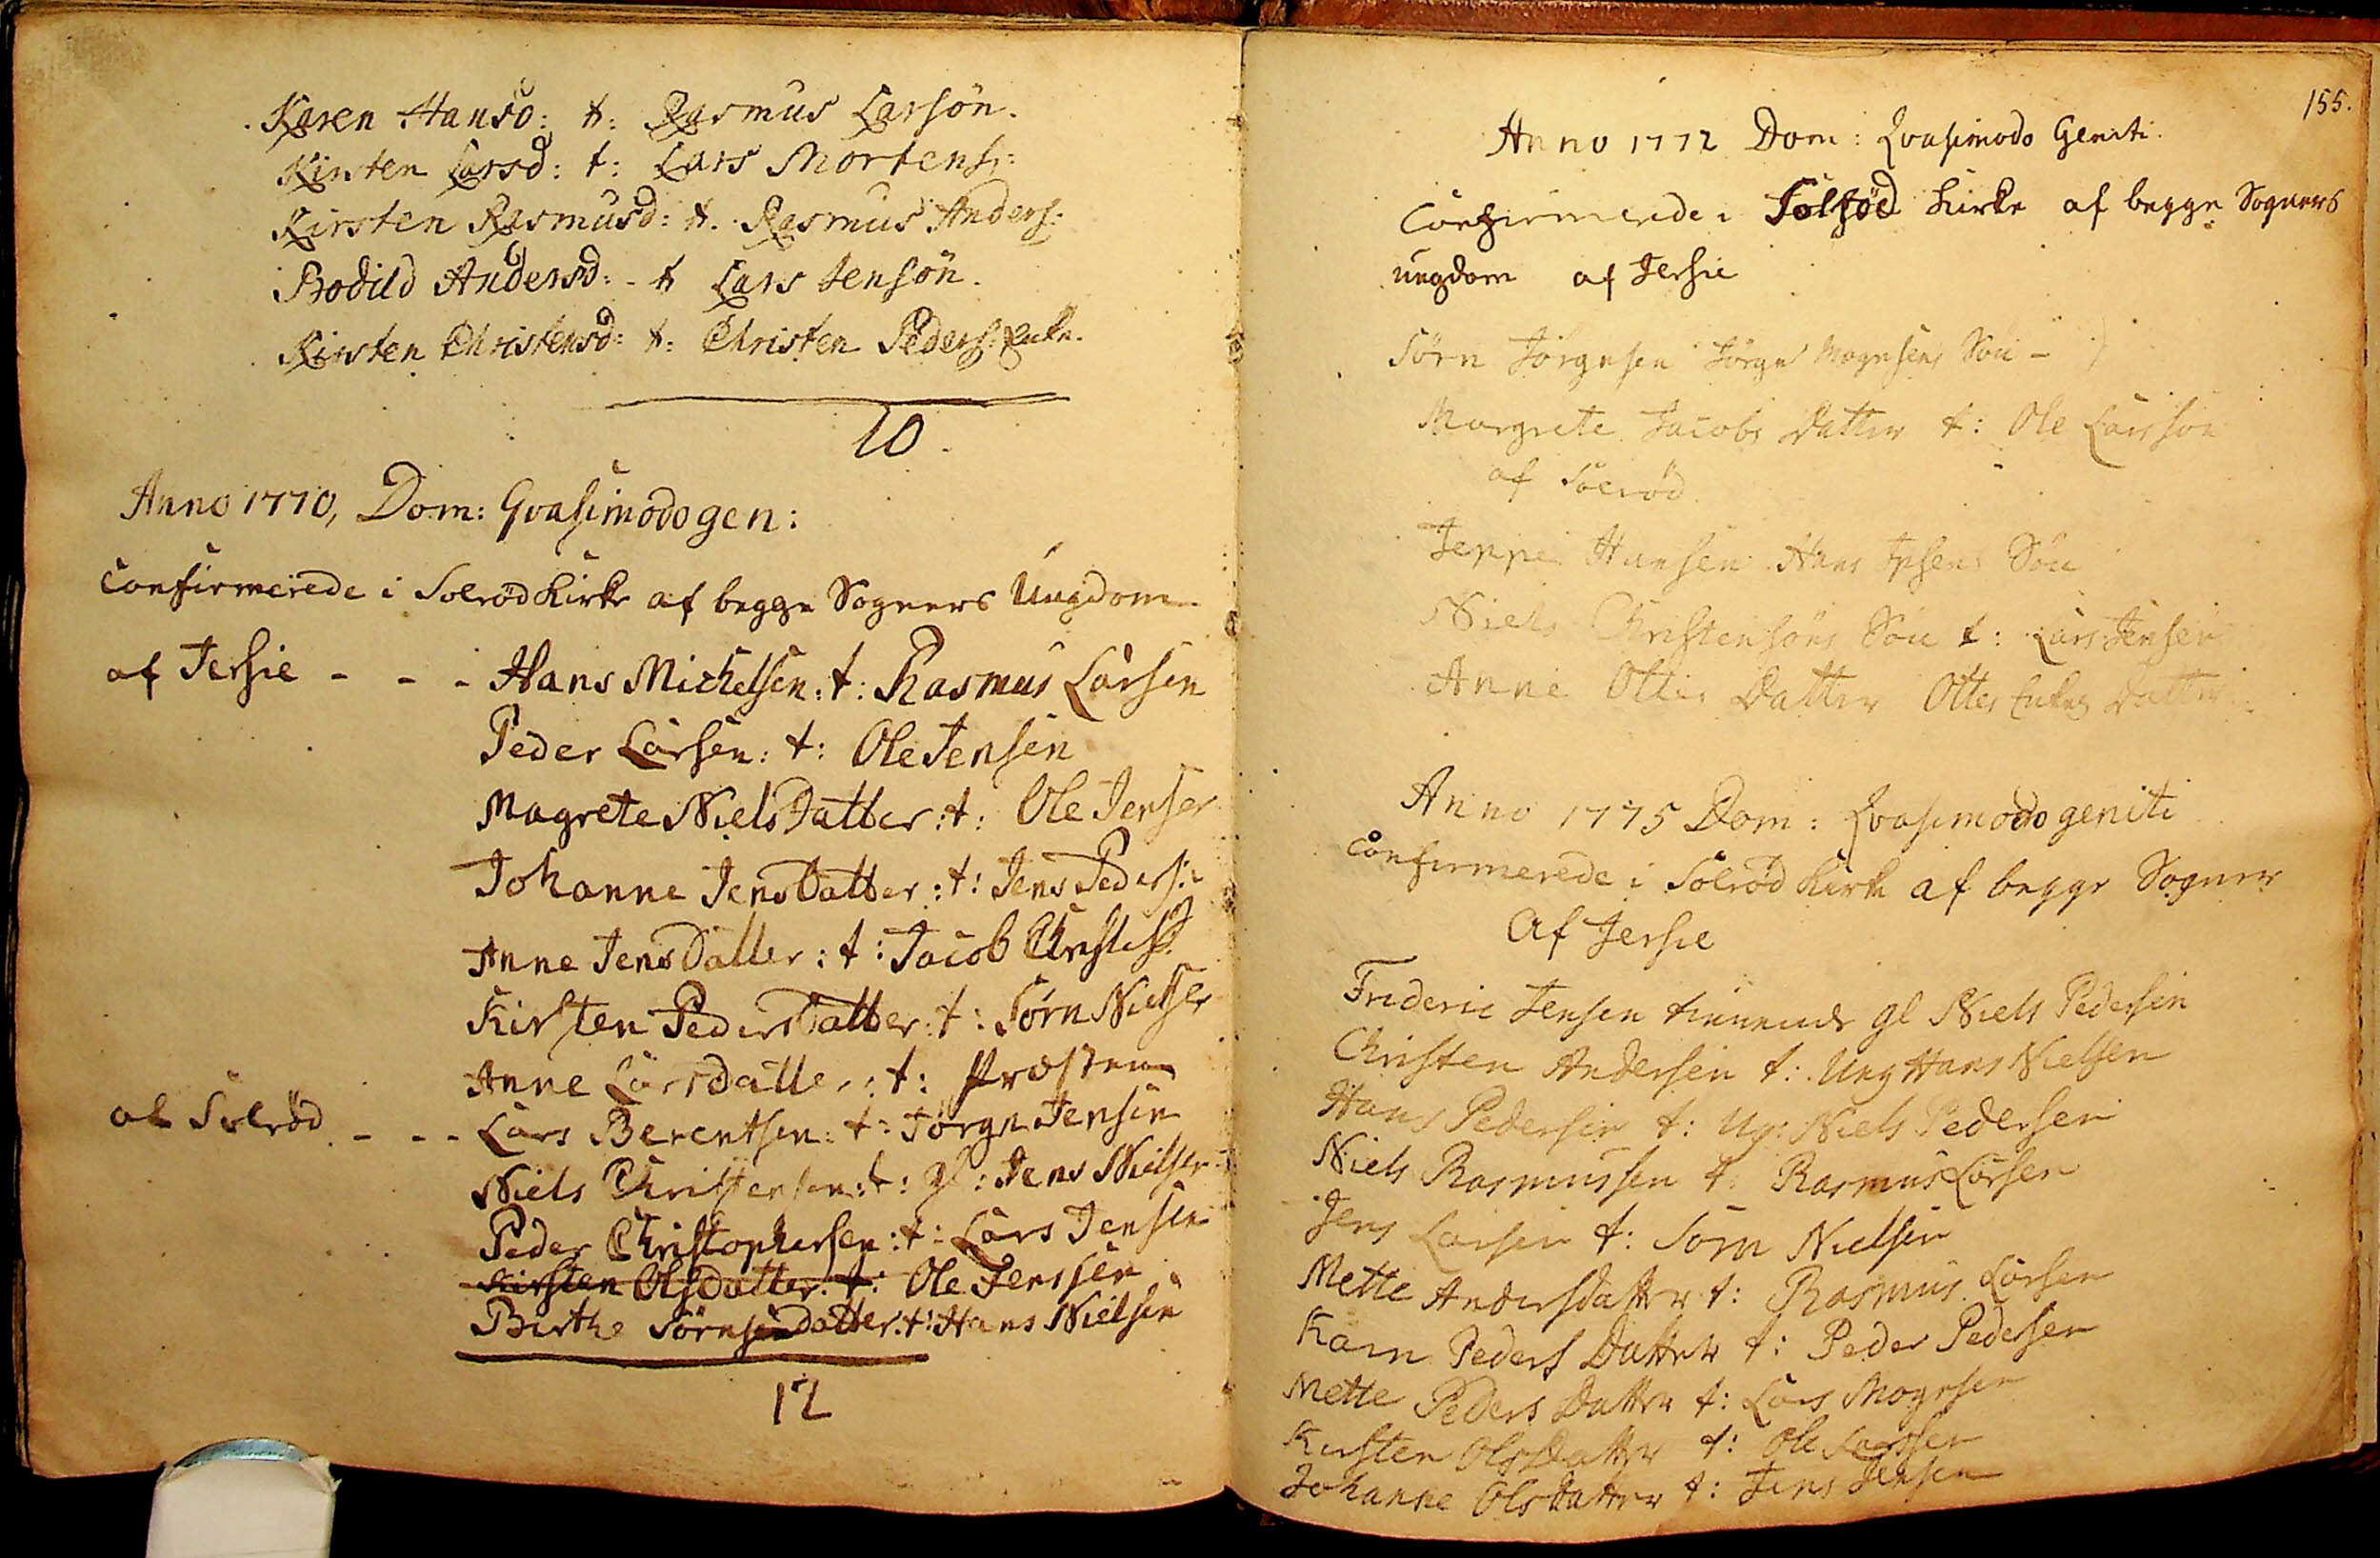Karen Hansd: t: Rasmus Larsøn.
Kirsten Larsd: t: Lars Mortens:
Kirsten Rasmusd: t. Rasmus Anders:
Bodild Andersd: t Lars Jensøn.
Kirsten Christensd: t: Christen Peders: Enke
10.
Anno 1770, Dom: Qvasimodogen:
Confirmerede i Solrød Kirke af begge Sogners Ungdom.
af Jersie - - - Hans Michelsen: t: Rasmus Larsen
Peder Larsen: t: Ole Jensen
Magrete NielsDatter: t: Ole Jensen
Johanne JensDatter: t: Jens Peders:
Anne JensDatter: t: Jacob Christens:
Kirsten PedersDatter: t: Sørn Nielsen
Anne LarsDatter: t: Præsten
af Solrød - - - Lars Berentsen: t: Jørgn Jensen
Niels Christensen: t: gl: Jens Nielsen
Peder Christophersen: t: Lars Jensen
Kirsten OlsDatter: t: Ole Jenssen
Birthe SørnsenDatter: t: Hans Nielsen
12
155.
Anno 1772 Dom: Qvasimodo Geniti
Confirmerede i Solrød Kirke af begge Sogners
ungdom af Jersie
Sørn Jørgnsen Jørgn Mognsens Søn -
Margrete Jacobs Datter t: Ole Larssen
af Solrød
Jeppe Hansen Hans Ibsens Søn
Niels Christensøns Søn t: Lars Jensen
Anne Ottes Datter Ottes Enkes Datter
Anno 1775 Dom: Qvasimodo geniti
Confirmerede i Solrød Kirke af begge Sogner
Af Jersie
Frideric Jensen tienende gl Niels Pedersen
Christen Andersen t: Ung Hans Nielsen
Hans Pedersen t: Ug: Niels Pedersen
Niels Rasmussen t: Rasmus Larsen
Jens Larsen t: Sørn Nielsen
Mette Andersdatter t: Rasmus Larsen
Karn Peders Datter t: Peder Pedersen
Mette Peders Datter t: Lars Mognsen
Kirsten OlsDatter t: Ole Larssen
Johanne OlsDatter t: Jens Jensen
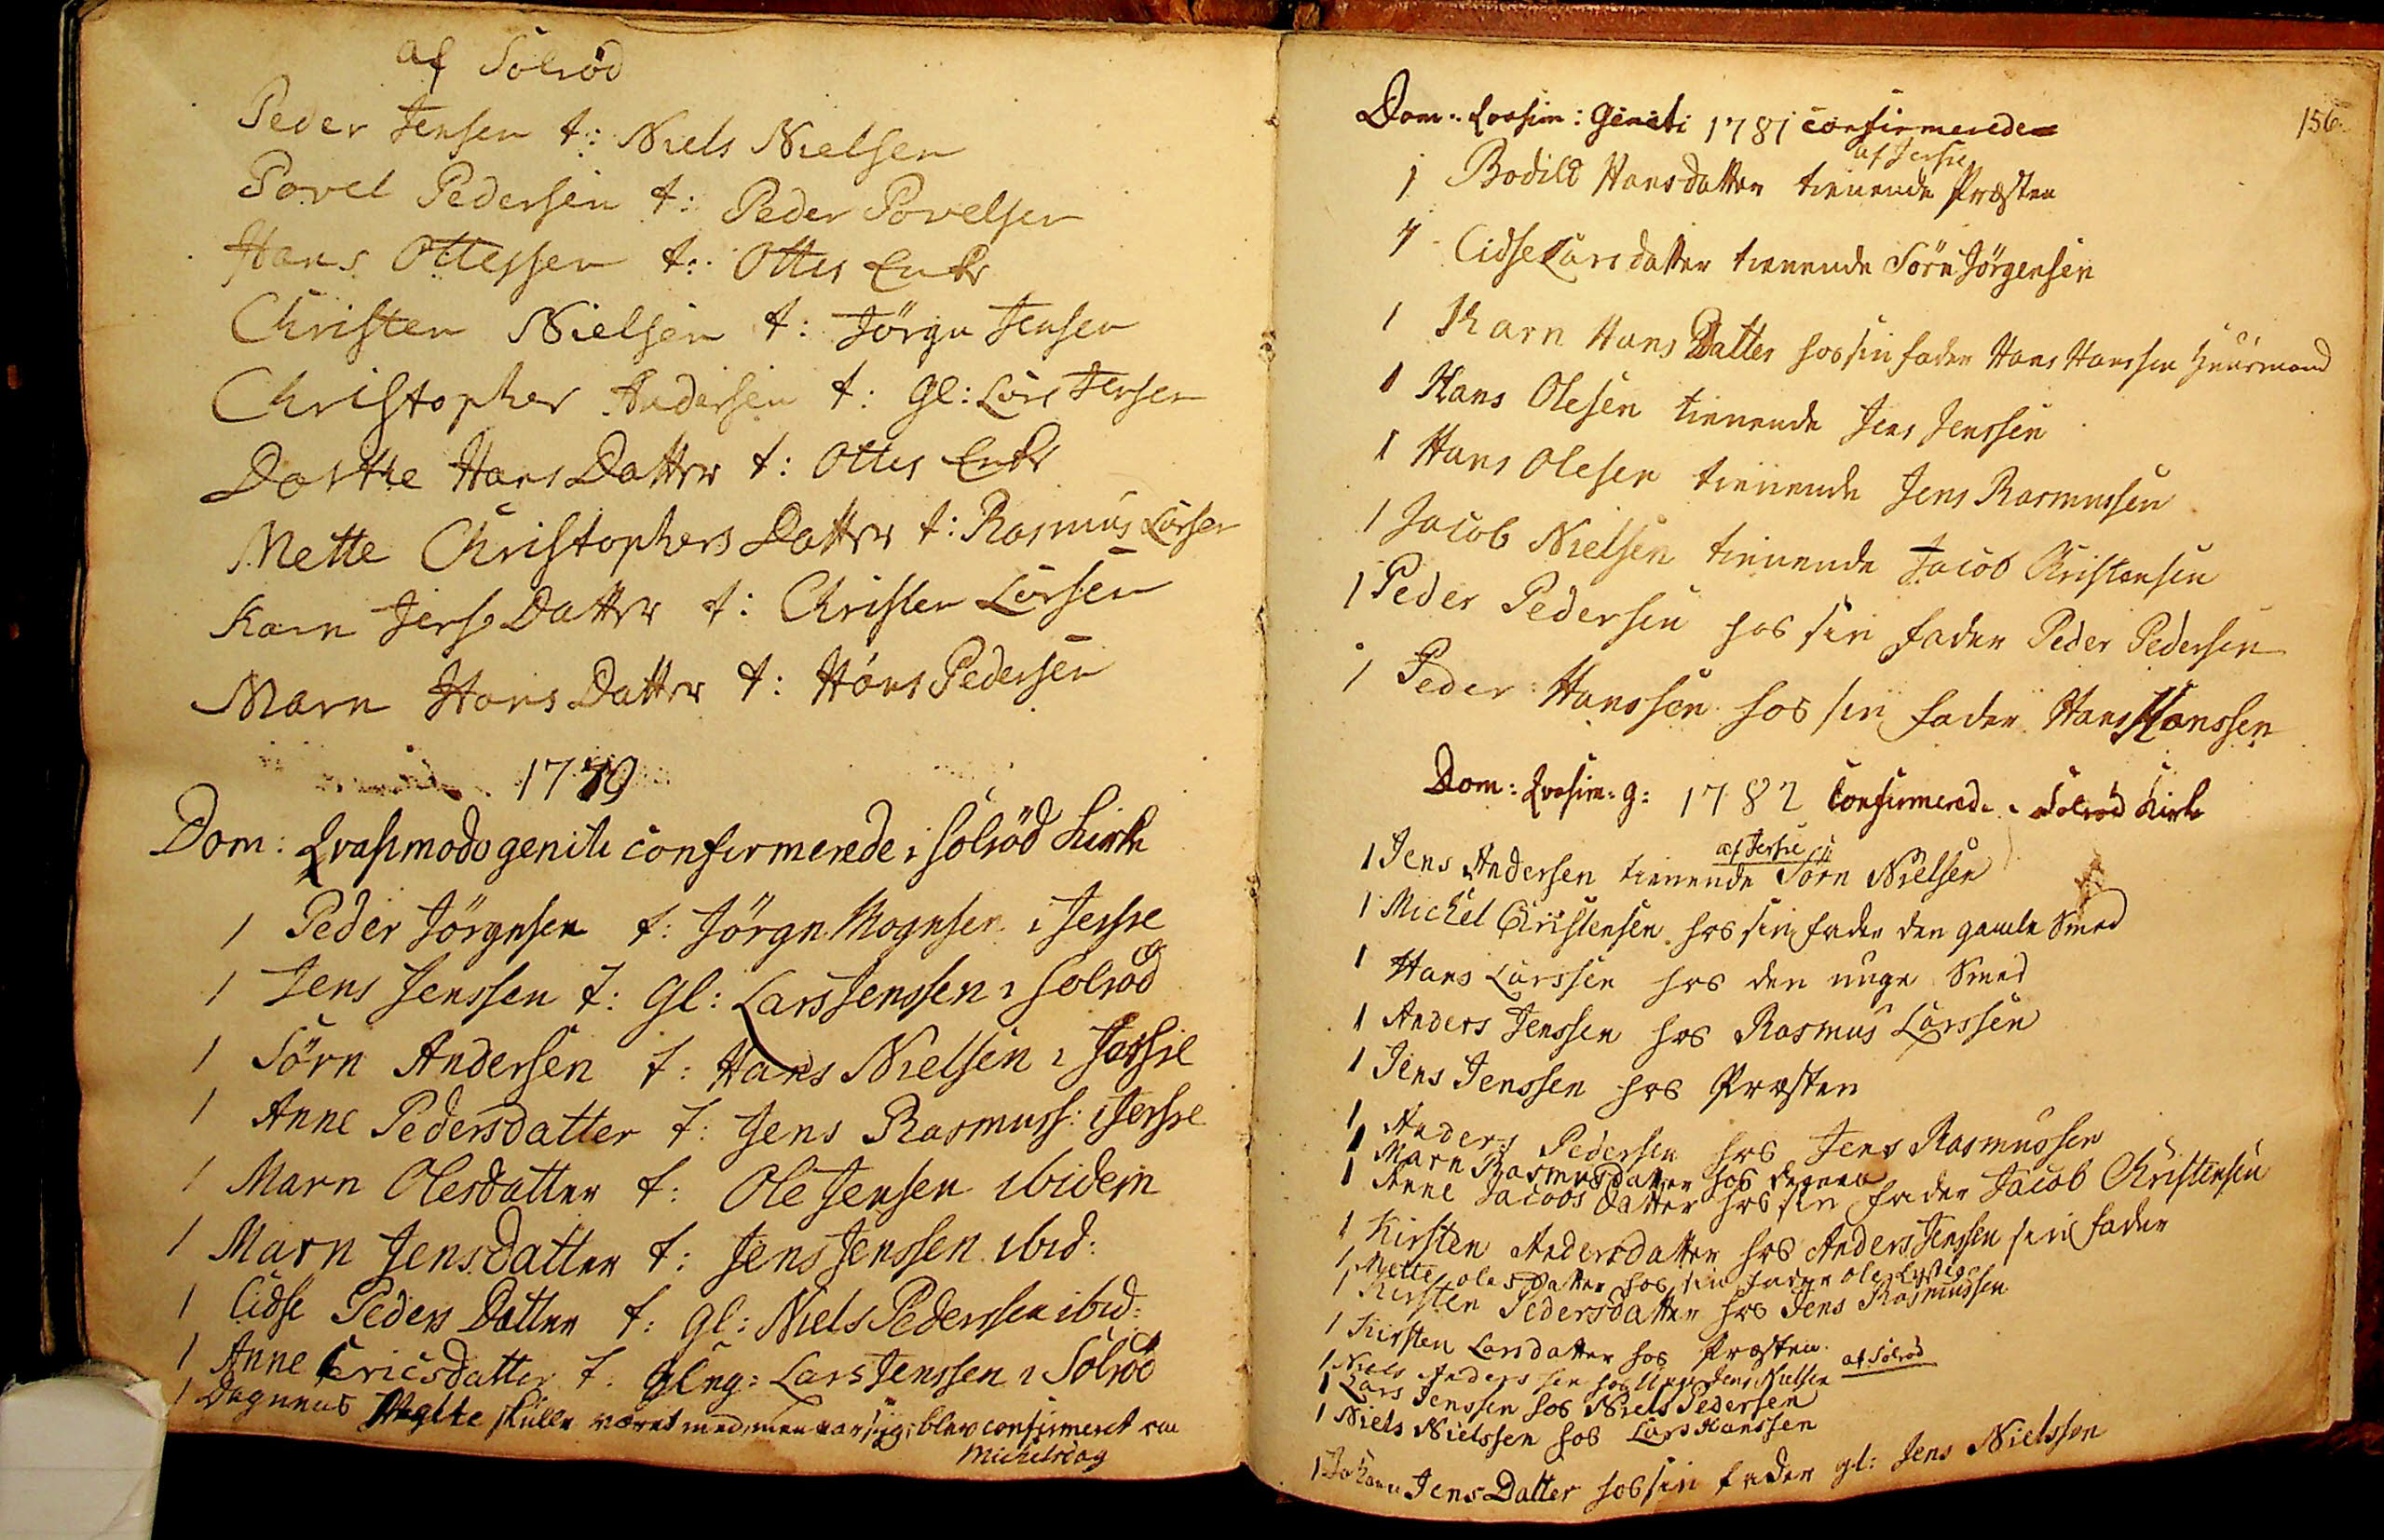af Solrød.
Peder Jensen t: Niels Nielsen
Povel Pedersen t: Peder Povelsen
Hans Ottessen t: Ottes Enke
Christen Nielsen t: Jørgn Jensen
Christopher Andersen t: gl: Lars Jensen
Dorthe Hans Datter t: Ottes Enke
Mette Christophers Datter t: Rasmus Larsen
Karn Jens Datter t: Christen Larsen
Marn Hans Datter t: Hans Pedersen
1779
Dom: Qvasimodo geniti confirmerede i Solrød Kirke
Peder Jørgensen t: Jørgn Mognsen i Jersie
Jens Jenssen t: Gl: Lars Jenssen i Solrød
Sørn Andersen t: Hans Nielsen i Jersie
Anne Pedersdatter t: Jens Rasmuss: i Jersie
Marn Olesdatter t: Ole Jensen ibidem
Marn Jensdatter t: Jens Jenssen ibid:
Cidse Peders Datter t: gl: Niels Pederssen ibid:
Anne Ericsdatter t: Ung: Lars Jenssen i Solrød
Degnens Mette skulle været med, men var syg; blev confirmeret sa##
Michelsdag
156.
Dom: Qvasim: Geniti 1781 confirmerede
af Jersie
Bodild Hansdatter tienende Præsten
Cidse Larsdatter tienende Sørn Jørgensen
Karn Hans Datter hos sin fader Hans Hansen Huusmand
Hans Olesen tienende Jens Jenssen
Hans Olesen tienende Jens Rasmussen
Jacob Nielsen tienende Jacob Christensen
Peder Pedersen hos sin Fader Peder Pedersen
Peder Hanssen hos sin Fader Hans Hanssen
Dom: Qvasim: g: 1782 Confirmerede i Solrød Kirke
af Jersie
Jens Andersen tienende Sørn Nielsen
Michel Christensen hos sin Fader den gamle Smed
Hans Larssen hos den unge Smed
Anders Jenssen hos Rasmus Larssen
Jens Jenssen hos Præsten
Anders Pedersen hos Jens Rasmussen
Marn Rasmusdatter hos Degnen
Anne Jacobsdatter hos sin Fader Jacob Christensen
Kirsten Andersdatter hos Anders Jenssen sin fader
Mette Olesdatter hos sin Fader Ole Lystig
Kirsten Pedersdatter hos Jens Rasmussen
Kirsten Larsdatter hos Præsten
af Solrød
Niels Anderssen hos Unge Jens Nielsen
Lars Jenssen hos Niels Pedersen
Niels Nielssen hos Lars Hanssen
Johane Jens Datter hos sin fader gl: Jens Nielssen